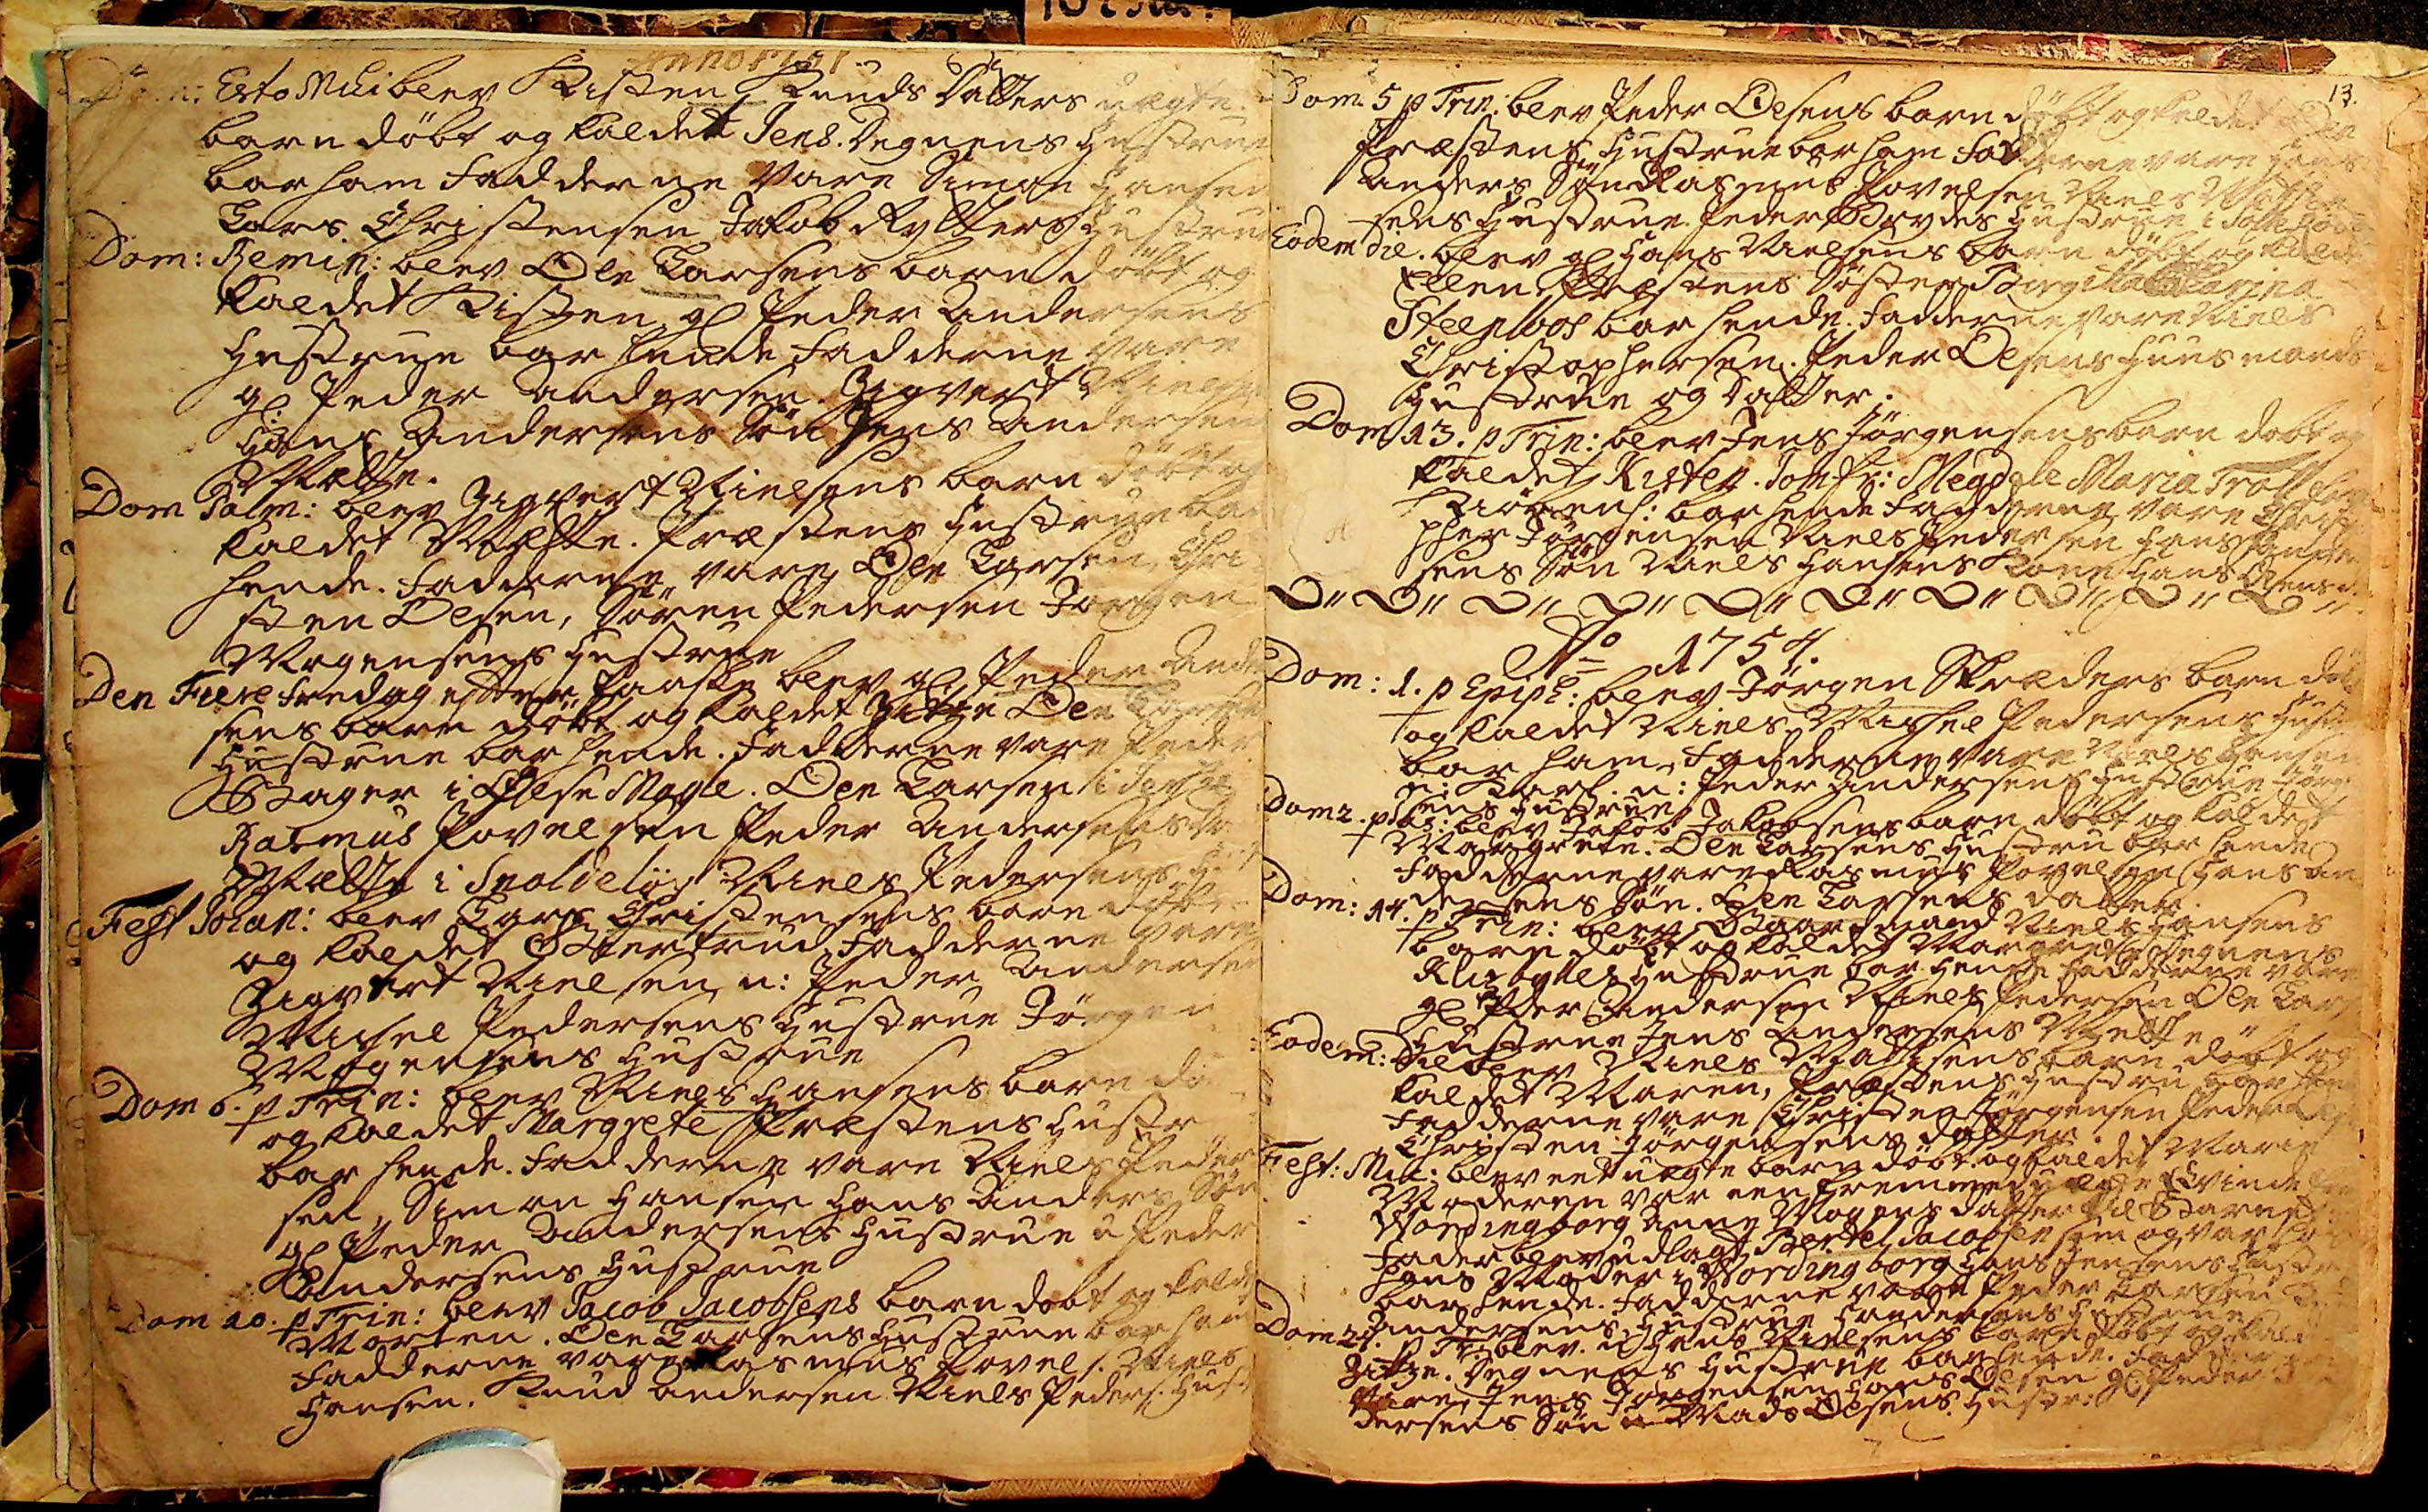Anno 1751
Dom: Esto Mihi blev Kirsten Knuds Datters uægte
barn døbt og kaldet Jens. Degnens Hustrue
bar ham Fadderne vare Simon Hansen
Lars Christensen Jacob Rytters Hustrue
Dom: Remin: blev Ole Larsens barn døbt og
kaldet Kisten gl Peder Andersens
Hustrue bar hende Fadderne vare
gl: Peder Andersen Zigvart Nielsen
Hans Andersens Søn Jens Andersens
Mætte.
Dom Palm: blev Zigvart Nielsens barn døbt og
kaldet Mætte. Præstens Hustrue bar
hende. Fadderne vare Ole Larsen, Chri¬
sten Olsen, Søren Pedersen Jørgen
Mogensens Hustrue
Den Fiere Søndag efter Paaske blev gl: Peder Ander
sens barn døbt og kaldet Zitze Ole Larsens
Hustrue bar hende. Fadderne vare Peder
Bager i Ølse Magle. Ole Larsen i Jersie
Rasmus Povelsen Peder Andersens Datter
Mætte i Snoldeløv Niels Pedersens Hust
Fest Johan: blev Lars Christensens barn døbt
og kaldet Giertrud Fadderne vare
Zigvart Nielsen u: Peder Andersen
Michel Pedersens Hustrue Jørgen
Mogensens Hustrue
Dom 6.p Trin: blev Niels Hansens barn døbt
og kaldet Margrete Præstens Hustrue
bar hende. Fadderne vare Niels Peder
sen, Simon Hansen  Hans Anders Søn
gl Peder Andersens Hustrue u Peder
Andersens Hustrue
Dom 10 p Trin: blev Jacob Jacobsens barn døbt og kaldet
Morten. Ole Larsens Hustrue bar ham
Fadderne vare Rasmus Povels. Niels
Hansen. Knud Andersen Niels Peders. Hustr
13.
Dom 5 p Trin: blev Peder Olsens barn døbt og kaldet Ole
Præstens Hustrue bar ham Fadderne vare Hans
Anders Søn Rasmus Povelsen Niels Mathie
sens Hustrue. Peder Brydes Hustrue i Solle Røde
Eodem Die. blev gl Hans Nielsens barn døbt og kaldet
Ellen. Præstens Søster Birgitta Catharina
Steenloos bar hende. Fadderne vare Niels 
Christophersen. Peder Olsens Huusmands
Hustrue og Datter.
Dom 13.p Trin: blev Jens Jørgensens barn døbt og
kaldet Kisten. Jomfr: Megdele Maria Troll fra
Kiøbenh: bar hende Fadderne vare Christo
pher Jørgensen Niels Pedersen Hans Ander
sens Søn Niels Hansens Kone Hans Olsens Dxx
Ao  1754.
Dom: 1. p Epiph: blev Jørgen Skræders barn døbt
og kaldet Niels  Michel Pedersens Hustr
bar ham. Fadderne vare Niels Hansen
u: Karl.  u: Peder Andersens Hustrue  Jørgen
sens Hustrue
Dom 2. p Pas: blev Jakob Jakobsens barn døbt og kaldet
Margrete. Ole Larsens Hustru bar hende
Fadderne vare Rasmus Povelsen Hans An
dersens Søn. Ole Larsens datter.
Dom: 14. p Trin: blev Gaardmand Niels Hansens
barn døbt og kaldet Margrete Degnens
Klizbylles Hustrue bar hende Fadderne vare
gl Peder Andersen Niels Pedersen Ole Lars.
Hustrue Jens Andersens Mette.
Eodem: Die blev Niels Mathsens barn døbt og
kaldet Maren, Præstens Hustru bar hende
Fadderne vare Christen Jørgensen Peder Olsen
Christen Jørgensens datter.
Fest Mik: blev eet uægte barn døbt og kaldet Maren
Moderen var een fremmed uægte Qvinde fra
Wordingborg Anne Mogens datter til Barnets
Fader blev udlagt Bertel Jacobsen som og var hos
hans Moder i Wordingborg Hans Jensens Hustrue
bar hende. Fadderne vare Peder Larsen Knud
Andersens Hustrue Handersens Hustrue
Dom 24. p Tr: blev u Hans Nielsens barn døbt og kaldet
Zitze. Degnens Hustrue bar hende Fadderne
vare Jens Jørgensen Hans Olsen gl Peder An
dersens Søn u Mads Olsens Hustr: